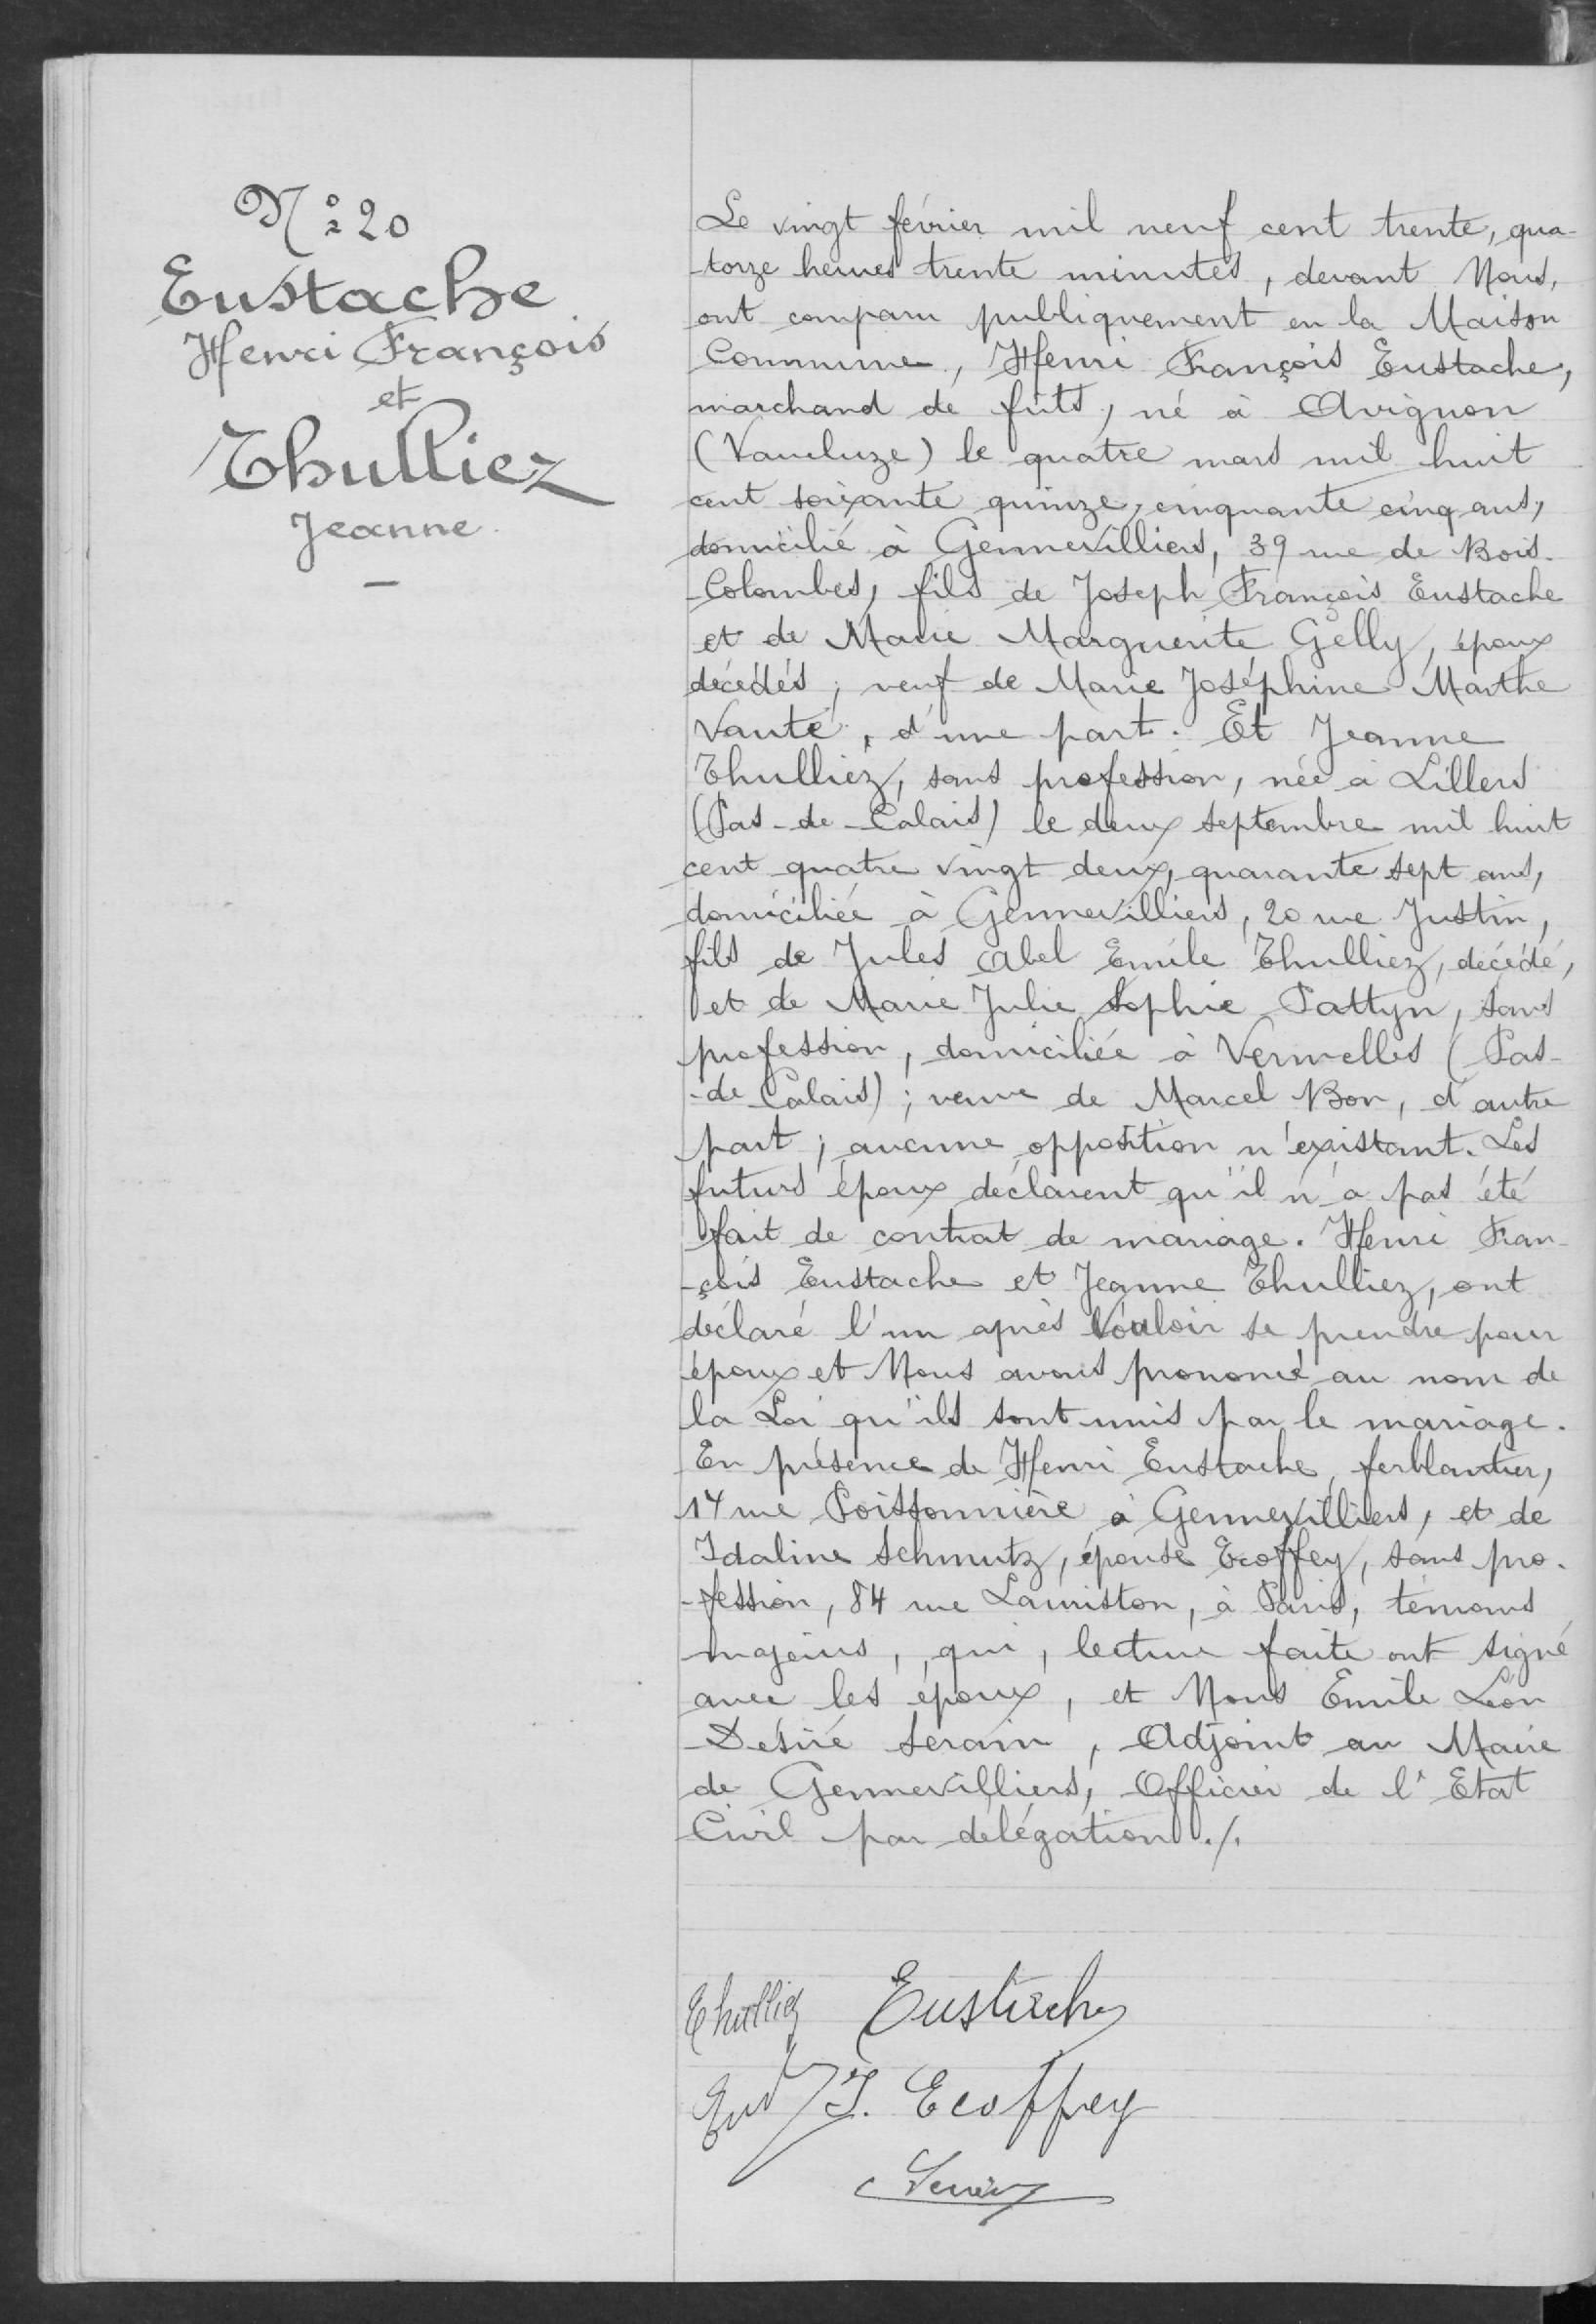N°20
Eustache
Henri François
et
Thullier
Jeanne
Le vingt février mil neuf cent trente, qua-
-torze heures trente minutes, devant Nous,
ont comparu publiquement en la Maison
Commune, Henri François Eustache,
marchand de fûts, né à Avignon
(Vaucluze) le quatre mars mil huit
cent soixante quinze, cinquante cinq ans,
domicilié à Gennevilliers, 39 rue de Bois-
-Colombes, fils de Joseph François Eustache
et de Marie Marguerite Gelly, époux
décédés, veuf de Marie Joséphine Marthe
Vante, d'une part. Et Jeanne
Thulliez, sans profession, née à Lilliers
(Pas-de-Calais) le deux septembre mil huit
cent quatre vingt deux, quarante sept ans,
domiciliée à Gennevilliers, 20 rue Justin,
fils de Jules Abel Emile Thulliez, décédé,
et de Marie Julie Sophie Pattyn, sans
profession, dommiciliée à Vermelles (Pas-
-de-Calais); veuve de Marcel Bron, d'autre
part; aucune opposition n'existant. Les
futurs époux déclarent qu'il n'a pas été
fait de contrat de mariage. Henri Fran-
-çois Eustache et Jeanne Thulliez, ont
déclaré l'un après vouloir se prendre pour
époux et Nous avons prononcé au nom de
la Loi qu'ils sont unis par le mariage.
En présence de Henri Eustache, ferblantier,
14 rue Poissonnière, à Genevilliers, et de
Idaline Schmitz, épouse Troffey, sans pro-
-fession, 84 rue Lamiston, à Paris, témoins
majeurs, qui, lecture faite ont signé
avec les époux, et Nous Emile Léon
Désiré Serain, Adjoint au Maire
de Gennevilliers, Officier de l'Etat
Civil par délégation ./.
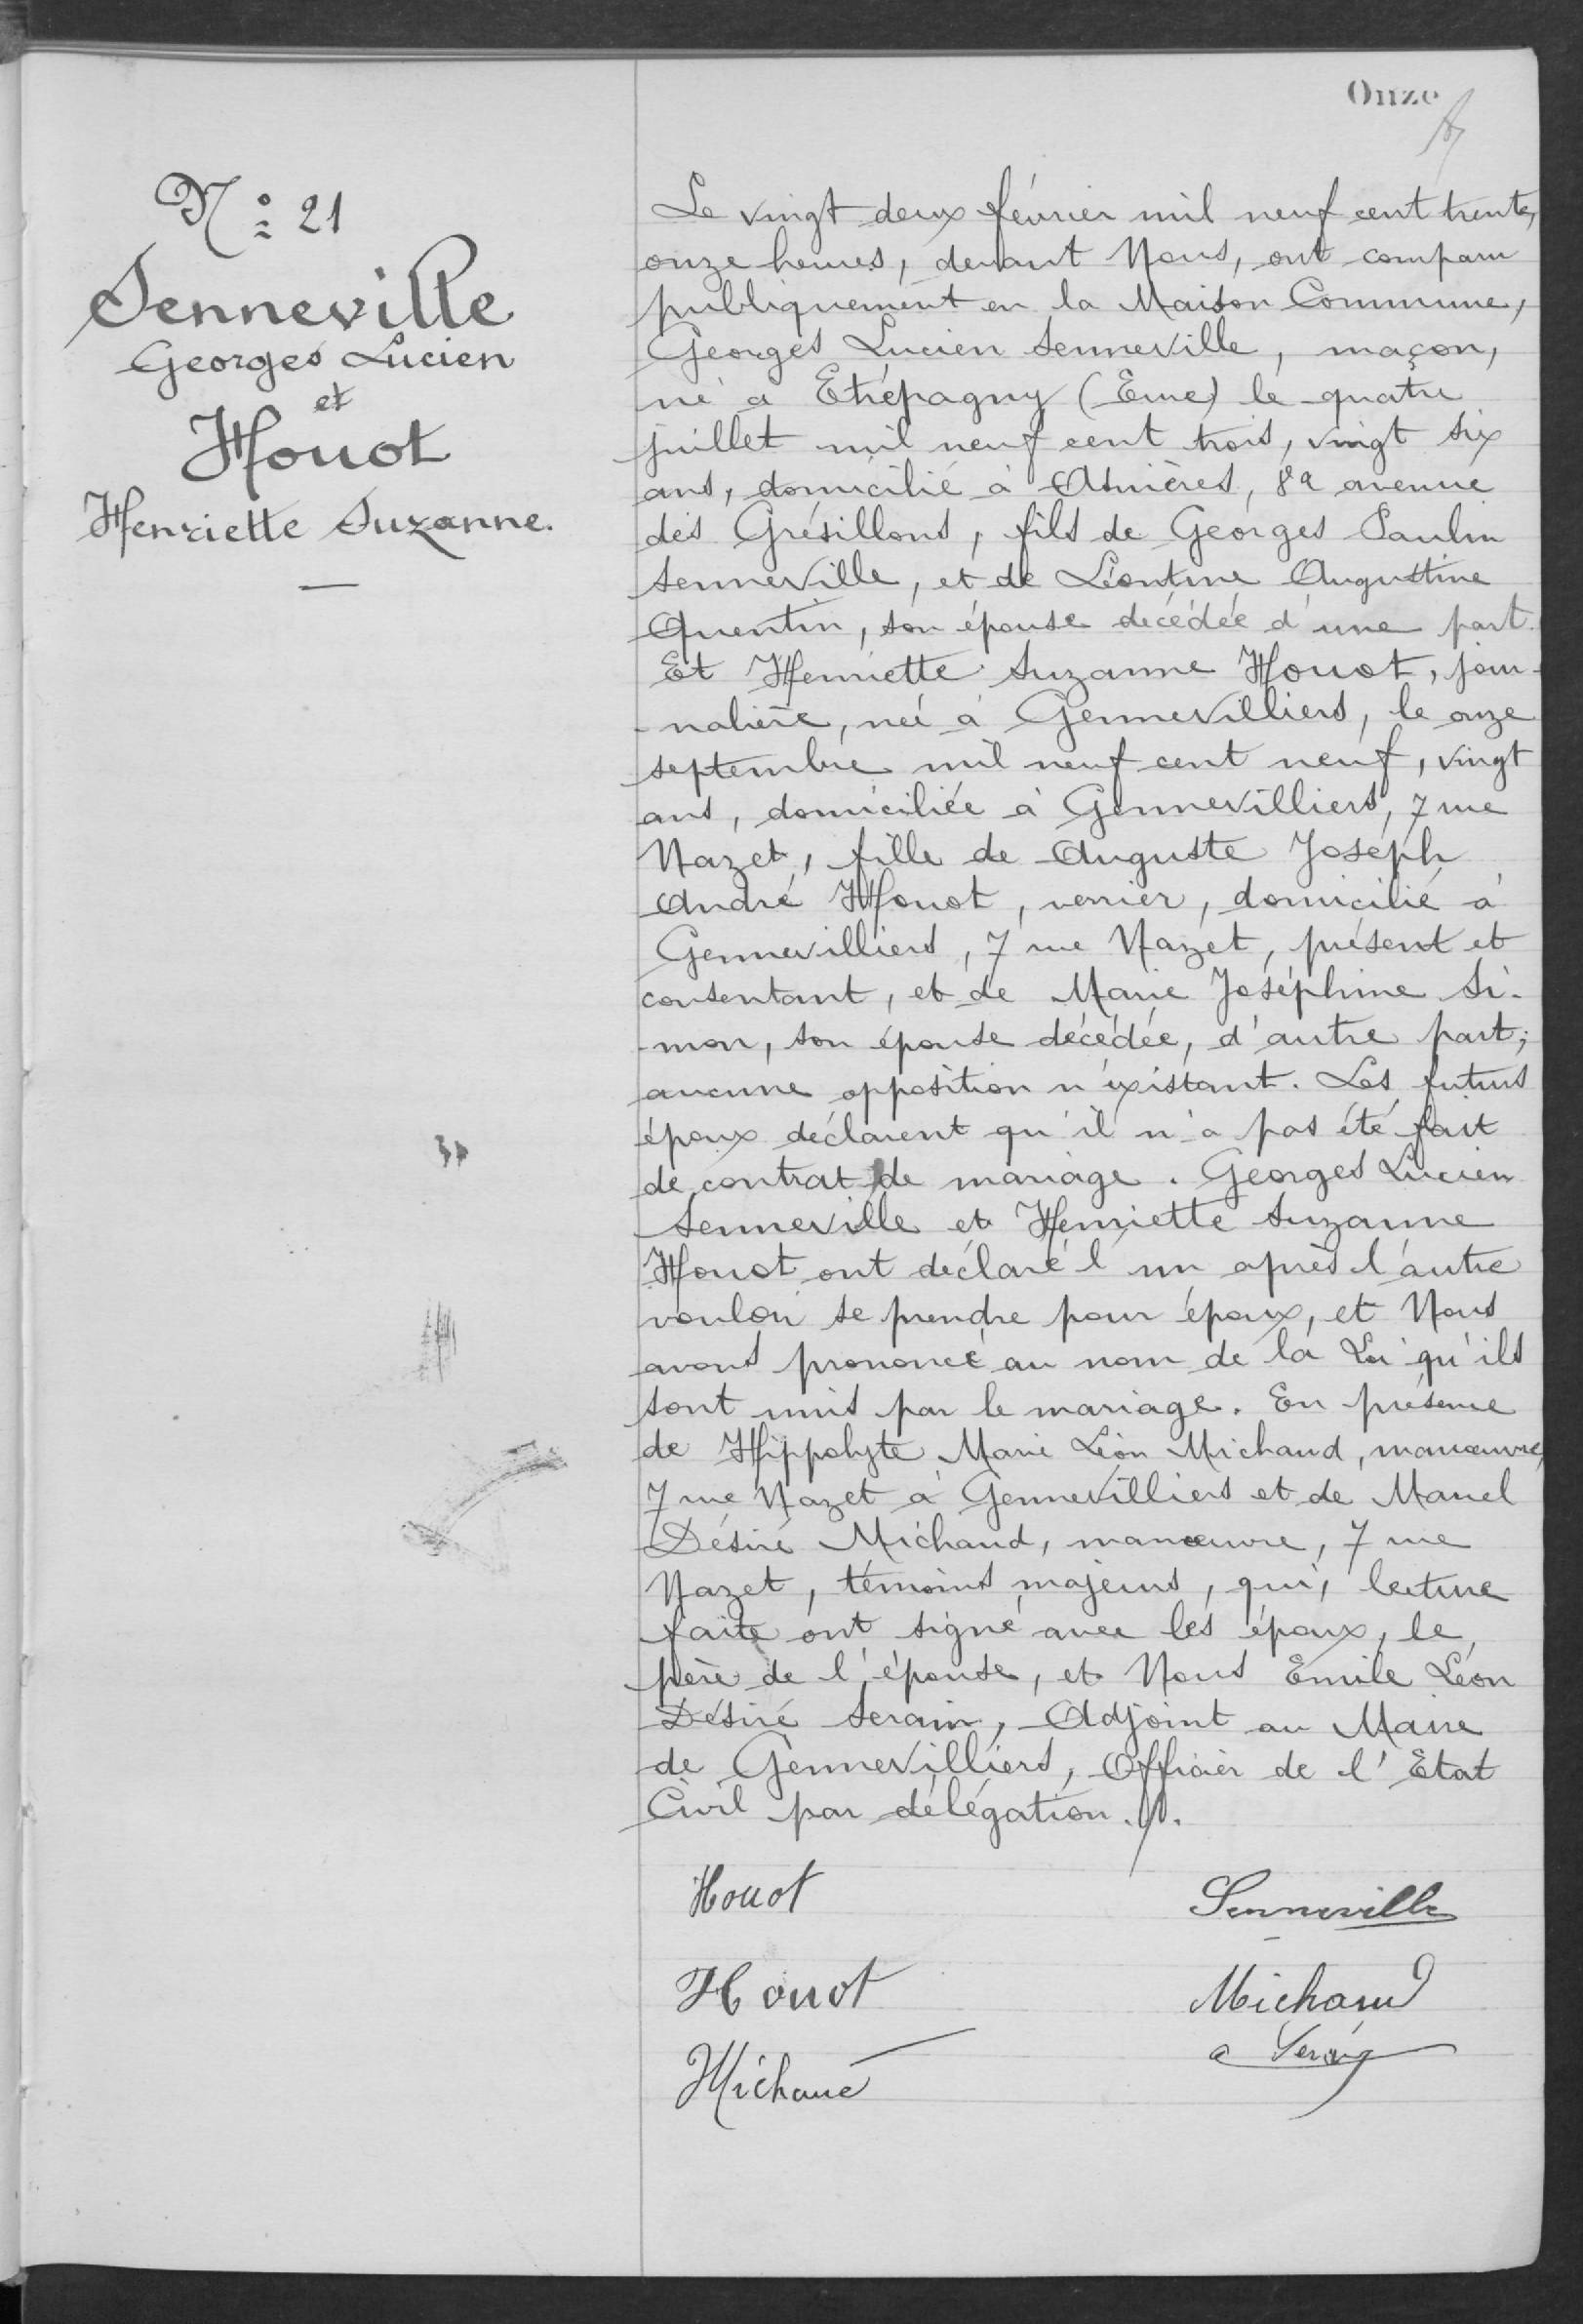N°21
Senneville
Georges Lucien
et
Houot
Henriette Suzanne
Le vingt deux février mil neuf cent trente,
onze heures, devant Nous, ont comparu
publiquement en la Maison Commune,
Georges Lucien Senneville, maçon,
né à Etrépagny (Erne) le quatre
juillet mil neuf cent trois, vingt six
ans, domicilié à Asnières, 89 avenue
des Grésillans, fils de Georges Paulin
Sennerville, et de Léontine Augustine
Quentin, son épouse décédée d'une part.
Et Henriette Suzanne Houot, jour-
-nalière, née à Gennevilliers, le onze
september mil neuf cent neuf, vingt
ans, domiciliée à Gennevilliers, 7 rue
Mazet, fille de Auguste Joseph
André Houot, verrier, domicilié à
Gennevilliers, 7 rue Mazet, présent et
consentant, et de Marie Joséphine Si-
-mon, son épouse décédée, d'autre part;
aucune opposition n'existant. Les futurs
époux déclarent qu'il n'a pas été fait
de contrat de mariage. Georges Lucien
Semmeville et Henriette Suzanne
Houot ont déclaré l'un après l'autre
vouloir se prendre pour époux, et Nous
avons prononcé au nom de la Loi qu'ils
sont unis par le mariage. En présence
de Hippolyte Marie Léon Michaud, manoeuvre,
7 rue Mazet, témoins majeurs, qui, lecture
fait ont signé avec les époux, le
père de l'épouse, et Nous Emile Léon
Désiré Serain, Adjoint au Maire
de Gennevilliers, Officier de l'Etat
Civil par délégation ./.